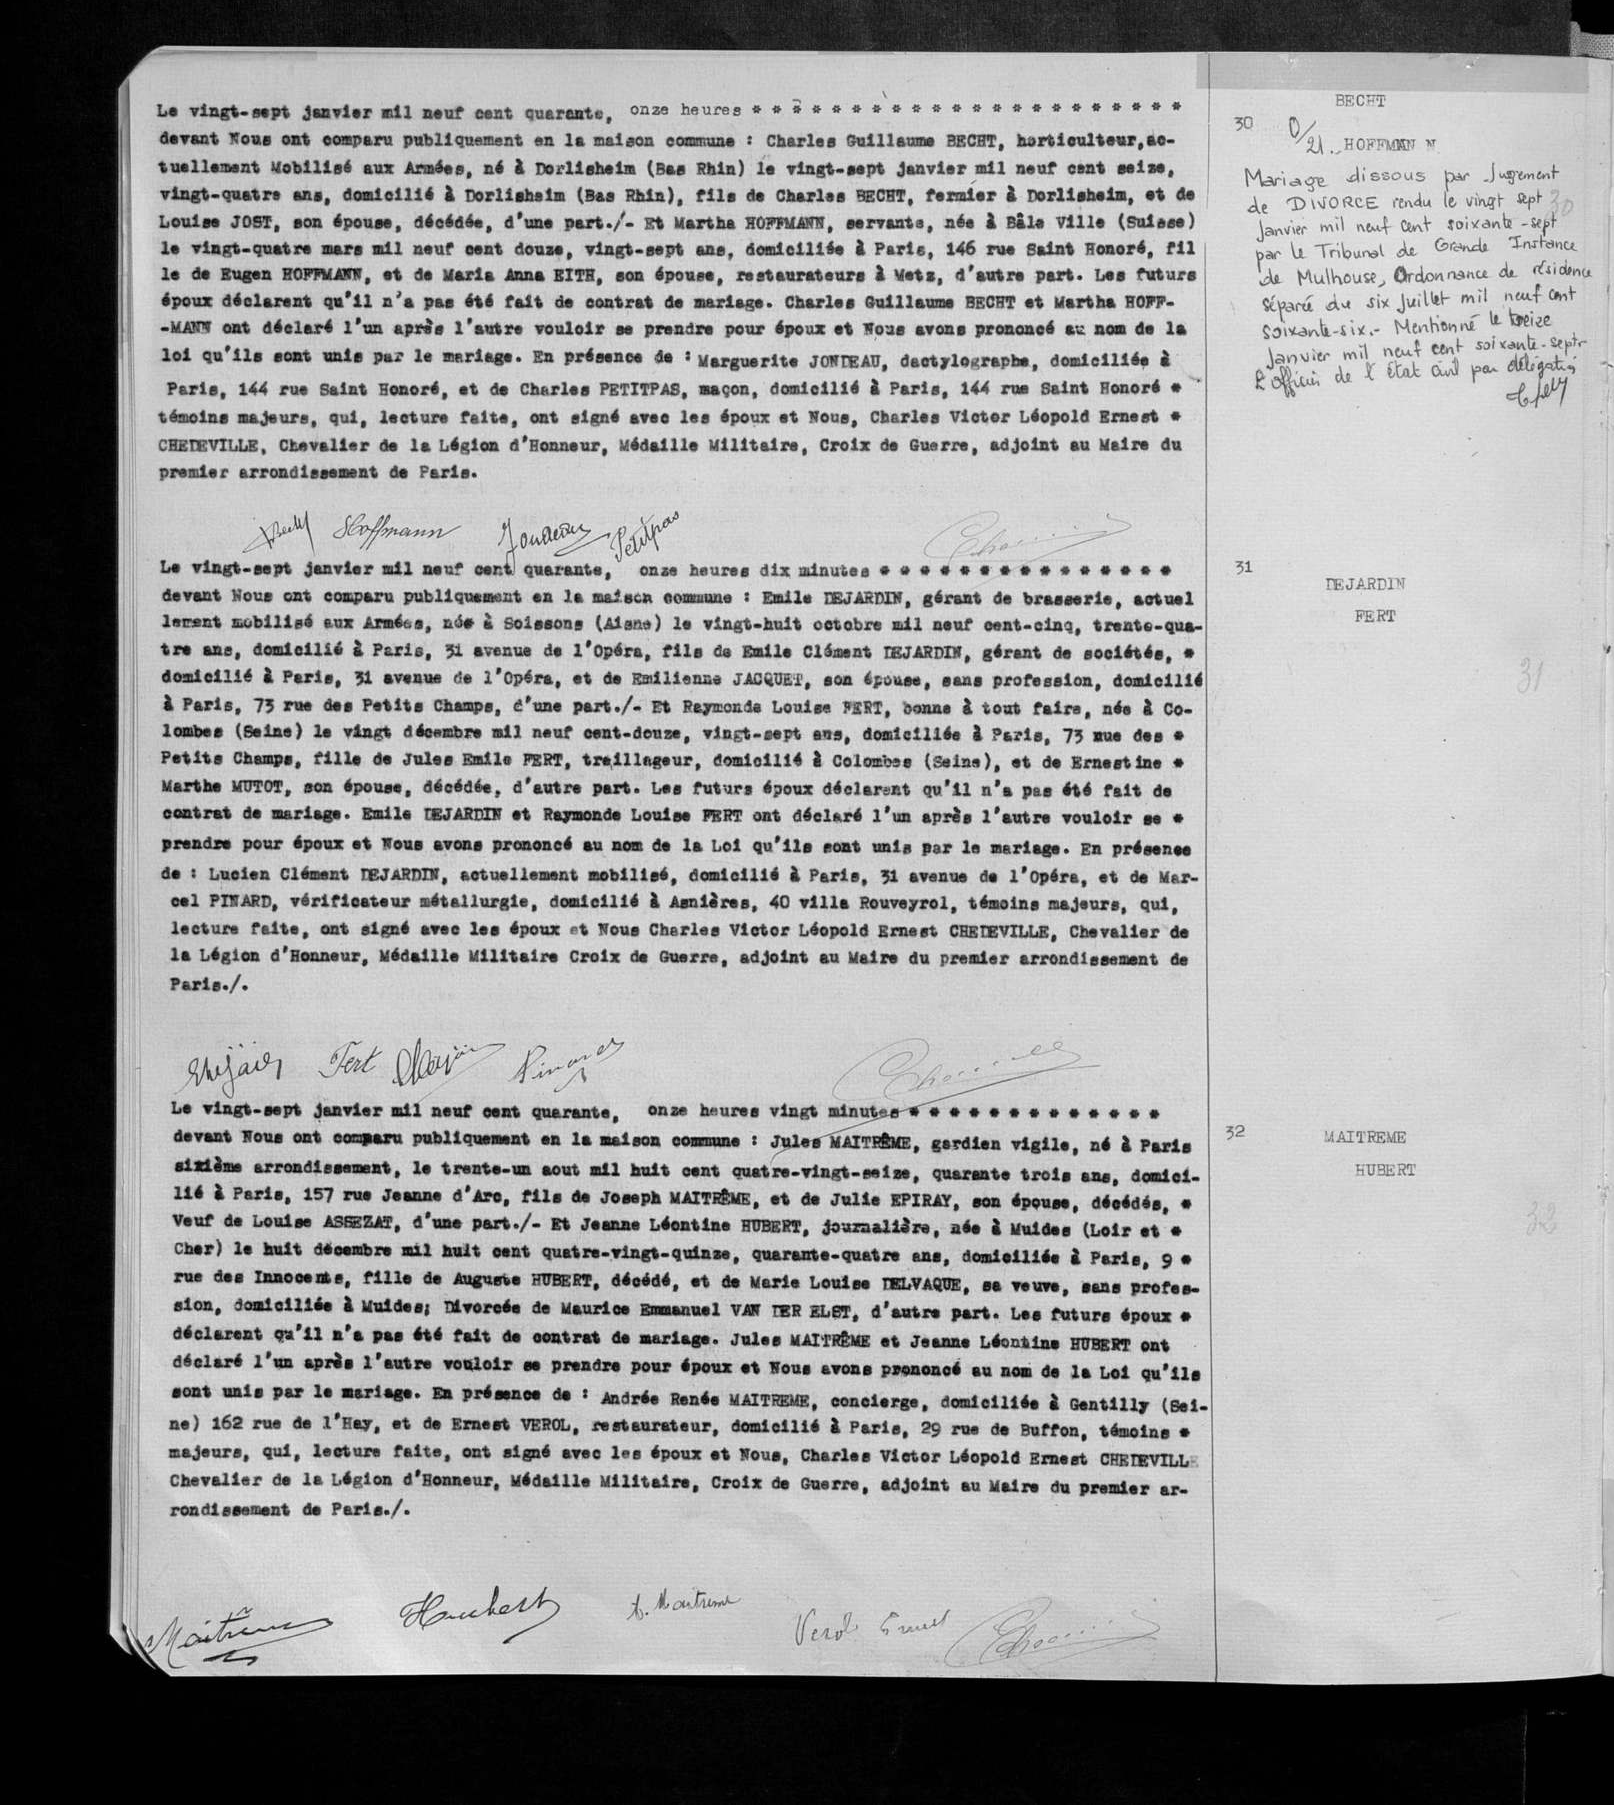Le vingt-sept janvier mil neuf cent quarante, onze heures ****
devant Nous ont comparu publiquement en la maison commune: Charles Guillaume BECHT, horticulteur, ac-
tuellement Mobilisé aux Armées, né à Dorlisheim (Bas Rhin) le vingt-sept janvier mil neuf cent seize,
vingt-quatre ans, domicilié à Dorlisheim (Bas Rhin), fils de Charles BECHT, fermier à Dorlisheim, et de
Louise JOST, son épouse, décédée, d'une part ./-Et Martha HOFFMAN, servants, née à Bâle Ville (Suisse)
le vingt-quatre mars mil neuf cent douze, vingt-sept ans, domiciliée à Paris, 146 rue Saint Honoré, fil
le de Eugen HOFFMAN, et de Maria Anna EITH, son épouse, restaurateurs à Metz, d'autre part. Les futurs
époux déclarent qu'il n'a pas été fait de contrat de mariage. Charles Guillaume BECHT et Martha HOFF-
-MANN ont déclaré l'un après l'autre vouloir se prendre pour époux et Nous avons prononcé au nom de la
loi qu'ils sont unis par le mariage. En présence de: Marguerite JONDEAU, dactylographe, domiciliée à
Paris, 144 rue Saint Honoré, et de Charles PETITPAS, maçon, domicilié à Paris, 144 rue Saint Honoré *
témoins majeurs, qui, lecture faite, ont signé avec les époux et Nous, Charles Victor Léopold Ernest *
CHELEVILLE, Chevalier de la Légion d'Honneur, Médaille Militaire, Croix de Guerre, adjoint au Maire du
premier arrondissement de Paris.
Le vingt-sept janvier mil neuf cent quarante, onze heures dix minutes ****
devant Nous ont comparu publiquement en la maison commune: Emilie DEJARDIN, gérant de brasserie, actuel
Element mobilisé aux Armées, née à Soissons (Aisne) le vingt-juin octobre mil neuf cent-cinq, trente-qua-
tre ans, domicilié à Paris, 31 avenue de l'Opéra, fils de Emilie Clément DEJARDIN, gérant de sociétés, *
domicilié à Paris, 31 avenue de l'Opéra, fils de Emilienne JACQUET, son épouse, sans profession, domicilié
à Paris, 73 rue des Petits Champs, d'une part ./-Et Raymonde Louise FERT, bonne à tout faire, née à Co-
lombes (Seine) le vingt décembre mil neuf cent-douze, vingt-sept ans, domiciliée à Paris, 73 rue des *
Petits Champs, fille de Jules Emile FERT, treillageur, domicilié à Colombe (Seine), et de Ernestine *
Marthe MUTOT, son épouse, décédée, d'autre part. Les futurs époux déclarent qu'il n'a pas été
fait de contrat de mariage. Emile DEJARDIN et Raymonde Louise FERT ont déclaré l'un après l'autre vouloir se *
prendre pour époux et Nous avons prononcé au nom de la Loi qu'ils sont unis par le mariage. En présence
de: Lucien Clément DEJARDIN, actuellement mobilisé, domicilié à Paris, 31 avenue de l'Opéra, et de Mar-
cel PINARD, vérificateur métallurgie, domicilié à Agnières, 40 villa Rouveyrol, témoins majeurs, qui,
lecture faite, ont signé avec les époux et Nous Charles Victor Léopold Ernest CHELEVILLE, Chevalier de
la Légion d'Honneur, Médaille Militaire Croix de Guerre, adjoint au Maire du premier arrondissement
de Paris ./.
Le vingt-sept janvier mil neuf cent quarante, onze heures vingt minutes ****
devant Nous ont comparu publiquement en la maison commune: Jules MAITREME, gardien vigile, né à Paris
sixième arrondissement, le trente-un aout mil huit cent quatre-vingt-seize, quarante trois ans, domici-
lié à Paris, 157 rue Jeanne d'Arc, fils de Joseph MAITREME, et de Julie EPIRAY, son épouse, décédés, *
Veuf de Louise ASSEZAT, d'une part ./-Et Jeanne Léontine HUBERT, journalière, née à Muides (Loir et *
Cher) le huit décembre mil huit cent quatre-vingt-quinze, quarante-quatre ans, domiciliée à Paris, 9 *
rue des Innocents, fille de Auguste HUBERT, décédé, et de Marie Louise DELVAQUE, sa veuve, sans profes-
sion, domiciliée à Muides; Divorcée de Maurice Emmanuel VAN DER ELST, d'autre part. Les futurs époux *
déclarent qu'il n'a pas été fait de contrat de mariage. Jules MAITREME et Jeanne Léontine HUBERT ont
déclaré l'un après l'autre vouloir se prendre pour épous et Nous avons prononcé au nom de la Loi qu'ils
sont unis par le mariage. En présence de: Andrée Renée MAITREME, concierge, domiciliée à Gentilly (Sei-
ne) 162 rue de l'Ha, et de Ernest VEROL, restaurateur, domicilié à Paris, 29 rue de Buffon, témoins *
majeurs, qui, lecture faite, ont signé avec les époux et Nous, Charles Victor Léopold Ernest CHELEVILLE
Chevalier de la Légion d'Honneur, Médaille Militaire, Croix de Guerre, adjoint au Maire du premier ar-
rondissement de Paris ./.
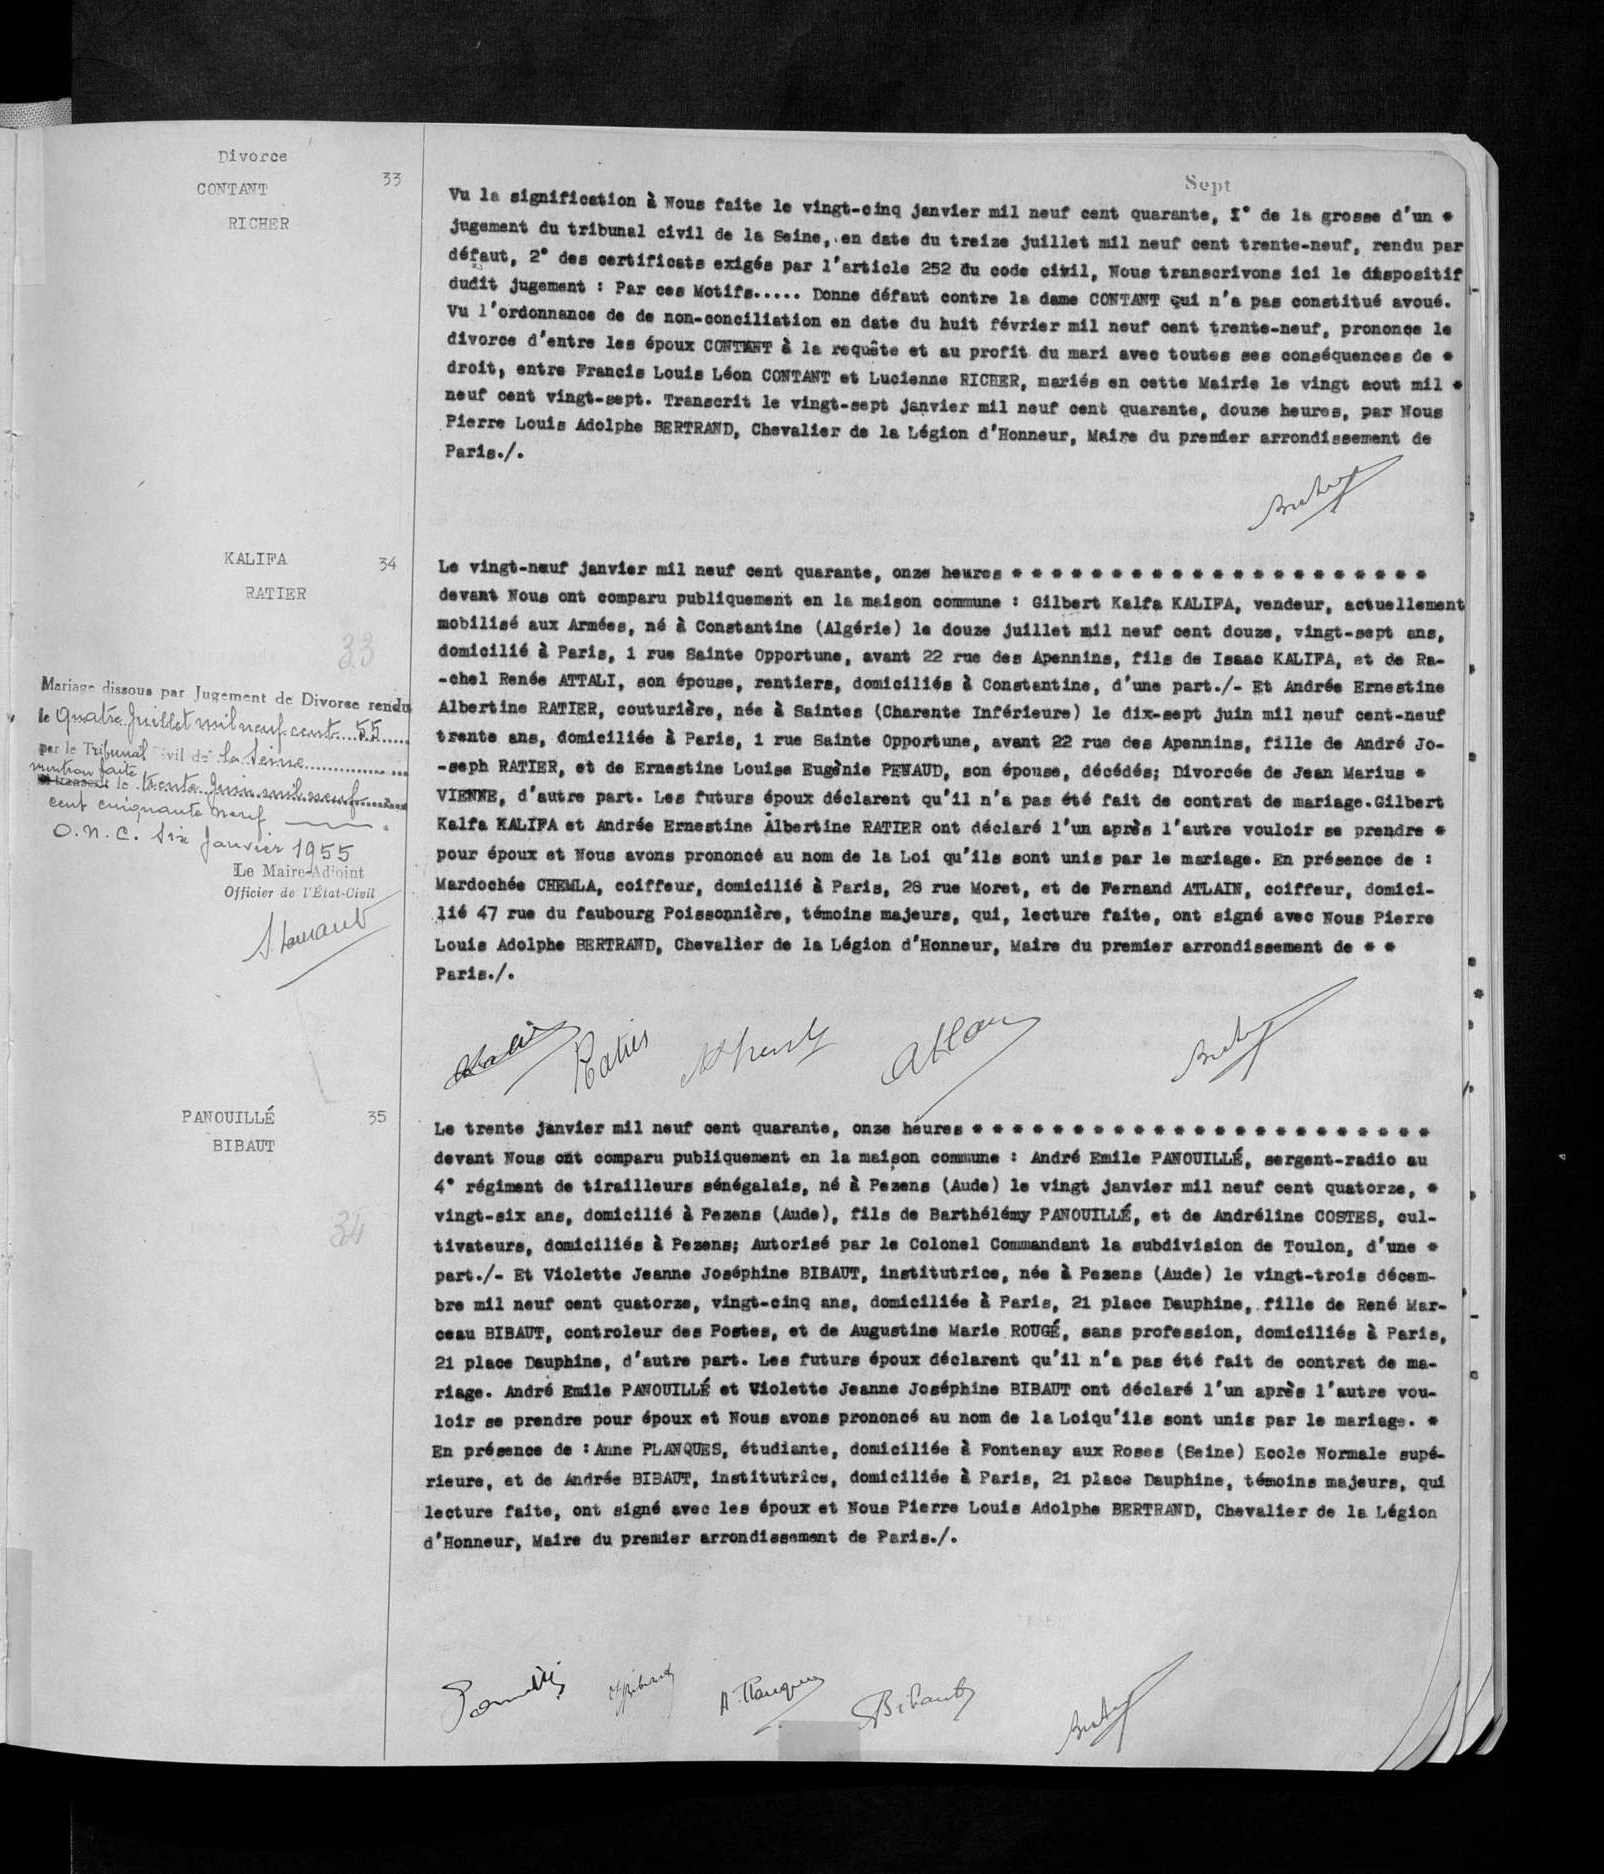Vu la signification à Nous faite le vingt-cinq janvier mil neuf cent quarante, I ° de la grosse
d'un * jugement du tribunal civil de la Seine, en date du treize juillet mil neuf cent trente-neuf, rendu par
défaut, 2° des certificats exigés par l'article 252 du code civil, Nous transcrivons ici le dispositif
dudit jugement: Par ces Motifs..... Donne défaut contre la dame CONTANT qui n'a pas constitué avoué.
Vu l'ordonnance de de non-conciliation en date du huit février mil neuf cent trente-neuf, prononce le
divorce d'entre les époux CONTANT à la requête et au profit du mari avec toutes ses conséquence de *
droit, entre francis Louis Léon CONTANT et Lucienne RICHER, mariés en cette Mairie le vingt aout mil *
neuf cent vingt-sept. Transcrit le vingt-sept janvier mil neuf cent quarante, douze heures, par Nous
Pierre Louis Adolphe BERTRAND, Chevalier de la Légion d'Honneur, Maire du premier arrondissement de
Paris ./.
Le vingt-neuf janvier mil neuf cent quarante, onze heures ****
devant Nous ont comparu publiquement en la maison commune: Gilbert Kalka KALIFA, vendeur actuellement
mobilisé aux Armées, né à Constantine (Algérie) le douze juillet mil neuf cent douze, vingt-sept ans,
domicilié à Paris, 1 rue Sainte Opportune, avant 22 rue des Apennins, fils de Isaac KALIFA, et de Ra-
-chel Renée ATTALI, son épouse, rentiers, domiciliées à Constantine, d'une part ./-Et Andrée Ernestine
Albertine RATIER, couturière, née à Saintes (Charente Inférieure) le dix-sept juin mil neuf cent-neuf
trente ans, domiciliée à Paris, 1 rue Sainte Opportune, avant 22 rue des Apennins, fille de André Jo-
-seph RATIER, et de Ernestin Louise Eugène PENAUD, son épouse, décédés; Divorcée de Jean Marius *
VIENNE, d'autre part. Les futurs époux déclarent qu'il n'a pas été fait de contrat de mariage. Gilbert
Kalfe KALIFA et Andrée Ernestine Albertine RATIER ont déclaré l'un après l'autre vouloir se prendre *
pour époux et Nous avons prononcé au nom de la Loi qu'ils sont unis par le mariage. En présence de:
Mardochée CHEMLA, coiffeur, domicilié à Paris, 29 rue Moret, et de Fernand ATLAIN, coiffure, domici-
lié 47 rue du faubourg Poissonière, témoins majeurs, qui, lecture faite, ont signé avec Nous Pierre
Louis Adolphe BERTRAND, Chevalier de la légion d'Honneur, Maire du premier arrondissement de ** Paris ./.
Le trente janvier mil neuf cent quarante, onze heures ****
devant Nous ont comparu publiquement en la maison commune: André Emile PANOUILLE, sergent-radio au
4° régiment de tirailleurs sénégalais, né à Pezens (Aude) le vingt janvier mil neuf cent quatorze, *
vingt-six ans, domicilié à Pezens (Aude), fils de Barthélémy PANOUILLE, et de Andréline COSTES, cul-
tivateurs, domiciliés à Pezens; Autorisé par le Colonel Commandant la subdivision de Toulon, d'une *
part ./-Et Violette Jeanne Joséphine BIBAUT, institutrice, née à Pezens (Aude) le vingt-trois décem-
bref mil neuf cent quatorze, vingt-cinq ans, domiciliée à Paris, 21 place Dauphine, fille de René Mar-
beau BIBAUT, controleur des Postes, et de Augustine Marie ROUGE, sans profession, domiciliés à Paris,
21 place Dauphine, d'autre part. Les futurs époux déclarent qu'il n'a pas été fait de contrat de ma-
riage. André Emile PANOUILLE et Violette Jeanne Joséphine BIBAUT ont déclaré l'un après l'autre vou-
loir se prendre pour époux et Nous avons prononcé au nom de la Loi qu'ils sont unis par le mariage .*
En présence de: Anne PLANQUES, étudiante, domiciliée à Fontenay aux Roses (Seine) Ecole Normale supé-
rieure, et de Andrée BIBAUT, institutrice, domiciliée à Paris, 21 place Dauphine, témoins majeurs, qui
lecture faite, ont signé avec les époux et Nous Pierre Louis Adolphe BERTRAND, Chevalier de la Légion
d'Honneur, Maire du premier arrondissement de Paris ./.